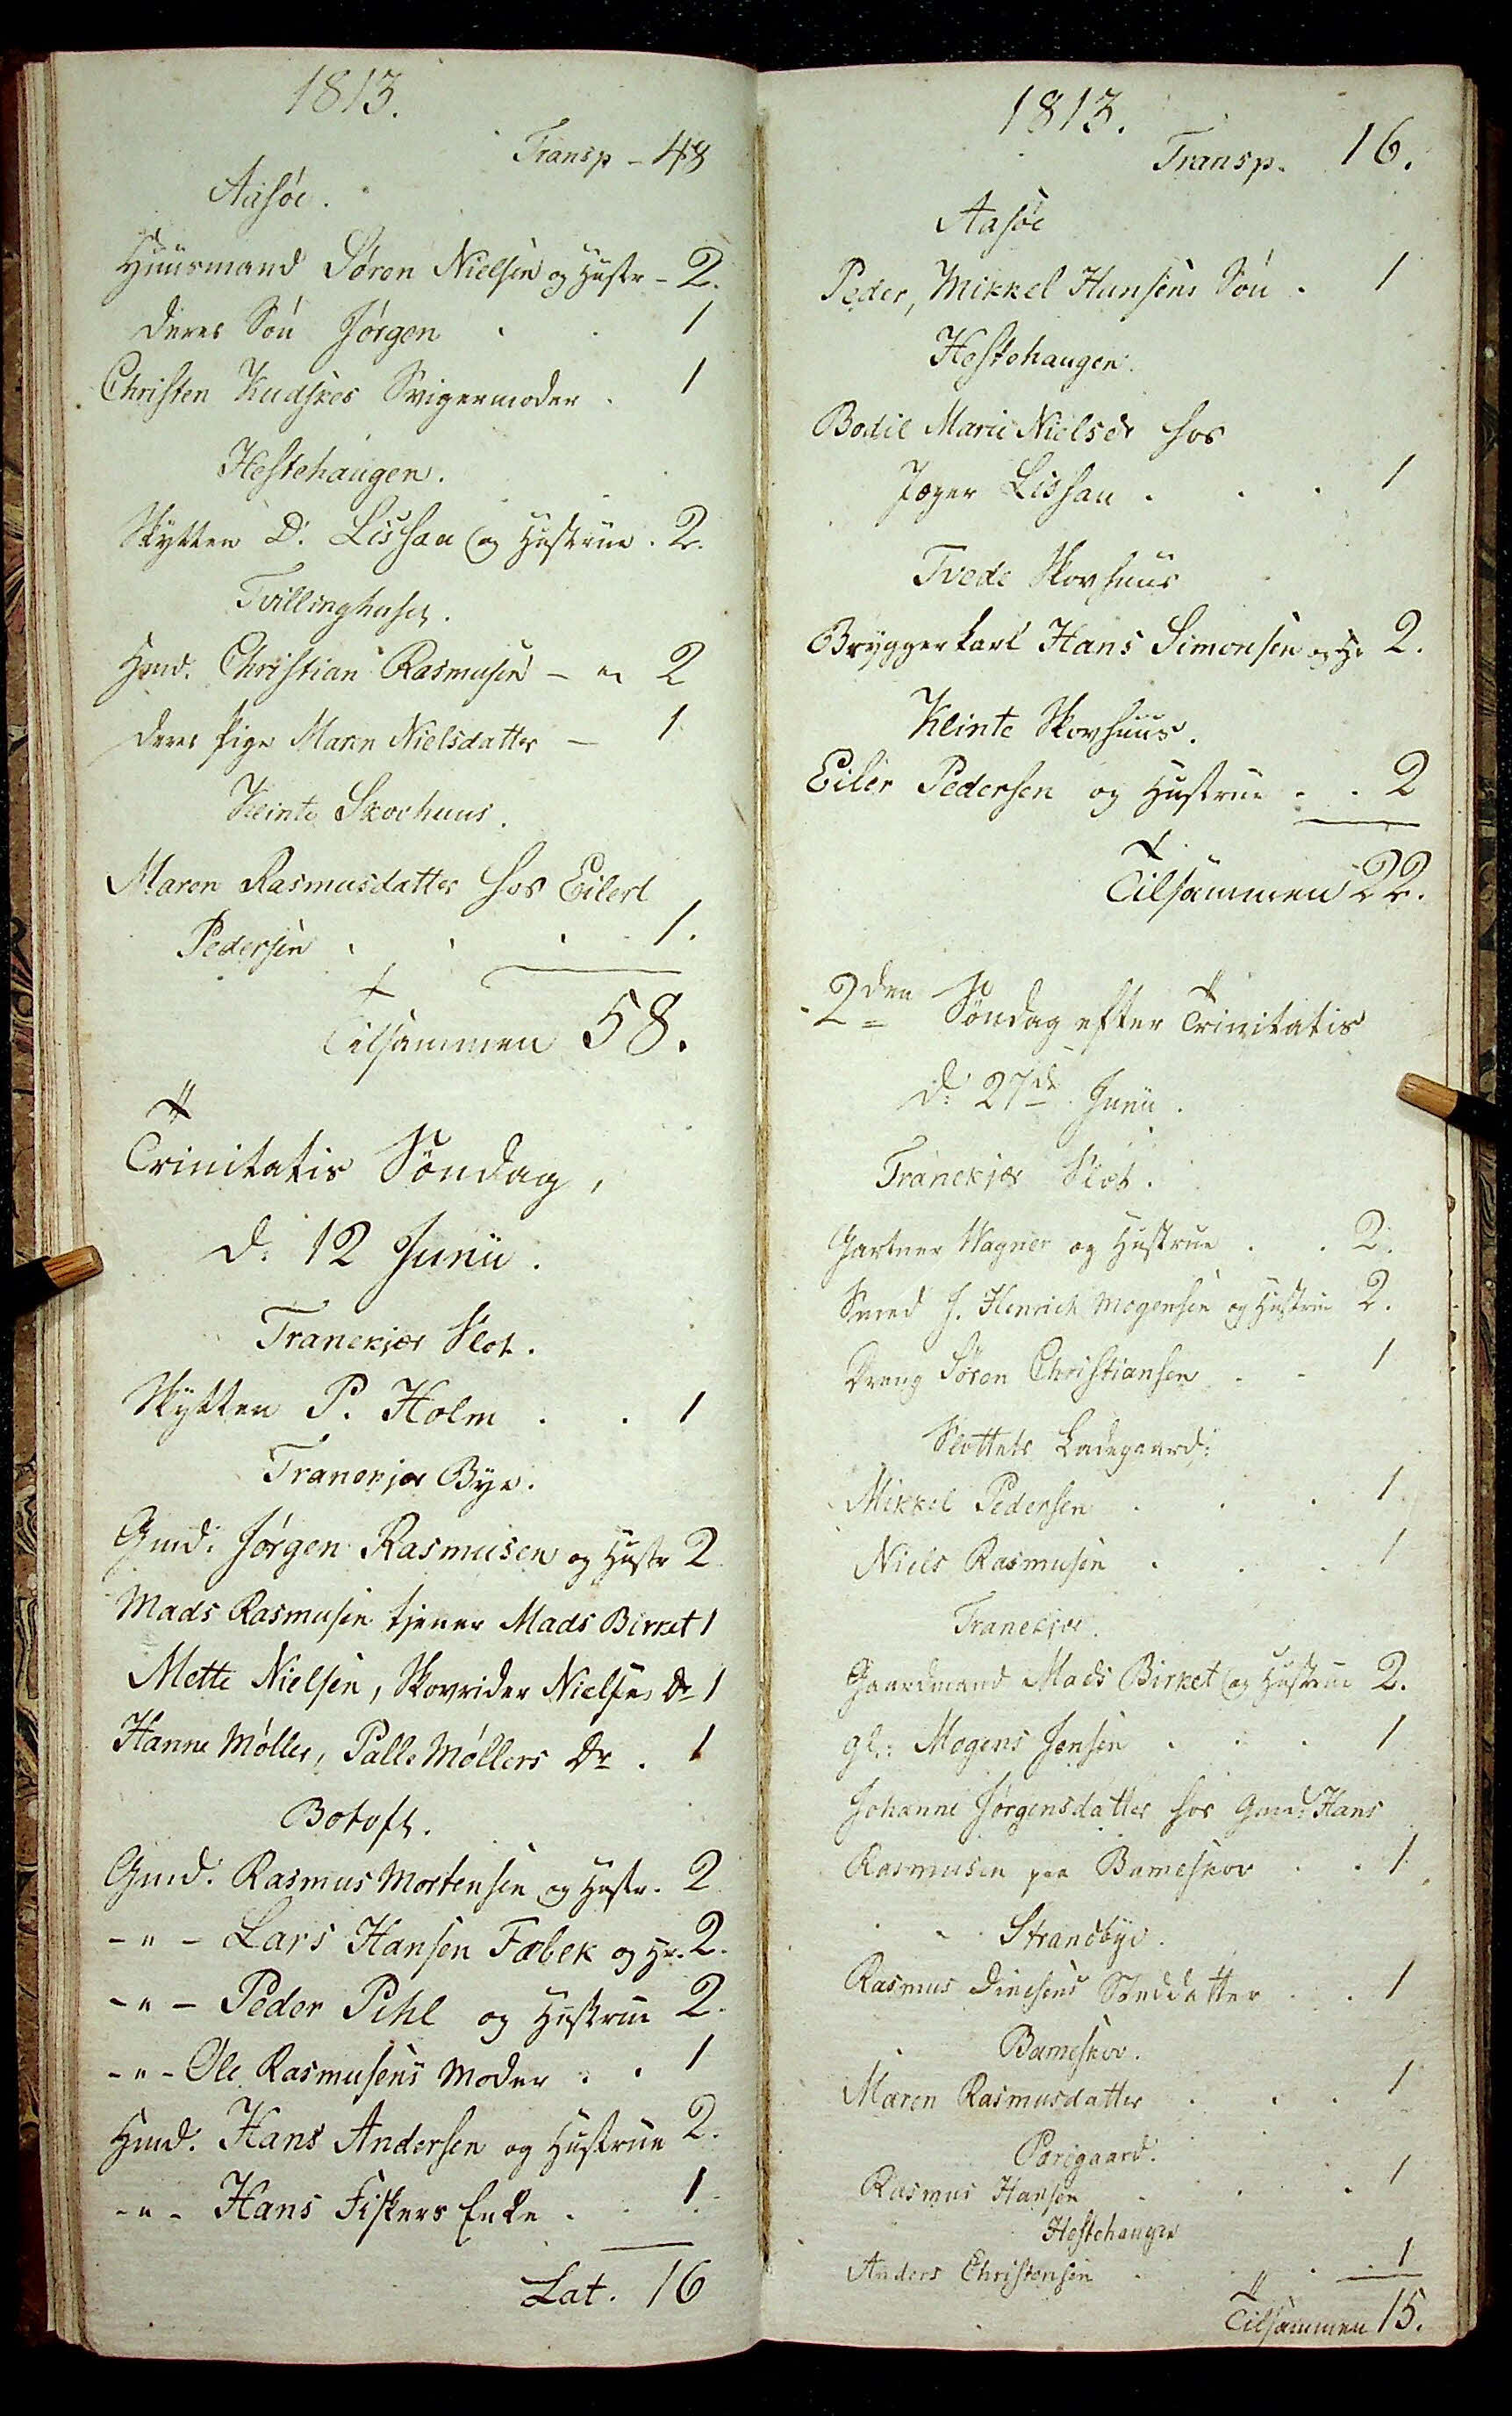1813.
Transp - 48
Aasøe
Huusmand Søren Nielsen og Hustr - 2.
deres Søn Jørgen . . 1
Christen Kudskes Svigermoder . 1
Hestehaugen.
Skytten D. Lissau og Hustrue . 2.
Tvillinghuset.
Hmd. Christian Rasmussen - - 2
deres Pige Maren Nielsdatter - 1
Klinte Skovhuus.
Maren Rasmusdatter hos Eilert
Pedersen . . . . 1.
Tilsammen 58.
Trinitatis Søndag,
d: 12 Junii.
Tranekjær Slot.
Skytten P. Holm . . 1
Tranekjær Bye.
Gmd. Jørgen Rasmusen og Hustr 2.
Mads Rasmusen tjener Mads Birret 1
Mette Nielsen, Skovrider Nielses Dr 1
Hanne Møller, Palle Møllers Dr . 1
Botoft.
Gmd. Rasmus Mortensen og Hustr . 2.
-"- Lars Hansen Fæbek og Hr . 2.
-"- Peder Pihl og Hustrue 2.
-"- Ole Rasmusens Moder . . 1
Hmd. Hans Andersen og Hustrue 2.
-"- Hans Fiskers Enke . . 1.
Lat. 16
1813.
Transp. 16.
Aasøe
Peder, Mikkel Hansens Søn . 1
Hestehaugen.
Bodil Marie Nielsdr hos
Jæger Lissau . . . 1
Tvede Skovhuus
Bryggerkarl Hans Simonsen og Hr 2.
Klinte Skovhuus
Eiler Pedersen og Hustrue . . 2
Tilsammen 22.
2den Søndag efter Trinitatis
d: 27de Junii
Tranekjær Slot.
Gartner Wagner og Hustrue . . 2
Smed J. Henrich Mogensen og Hustrue 2.
Dreng Søren Christiansen . . 1
Slottets Ladegaard:
Mikkel Pedersen . . . 1.
Niels Rasmussen . . . 1
Tranekjær
Gaardmand Mads Birket og Hustrue 2.
gl: Mogens Jensen . . . 1
Johanne Jørgensdatter hos Gmd: Hans
Rasmusen paa Bameskov . . 1
Strandbye.
Rasmus Dinesens Sønddatter . . 1
Bamesskov.
Maren Rasmusdatter . . . 1
Pæregaard.
Rasmus Hansøn . . . 1
Hestehaugen
Anders Christensen . . . . 1
Tilsammen 15.
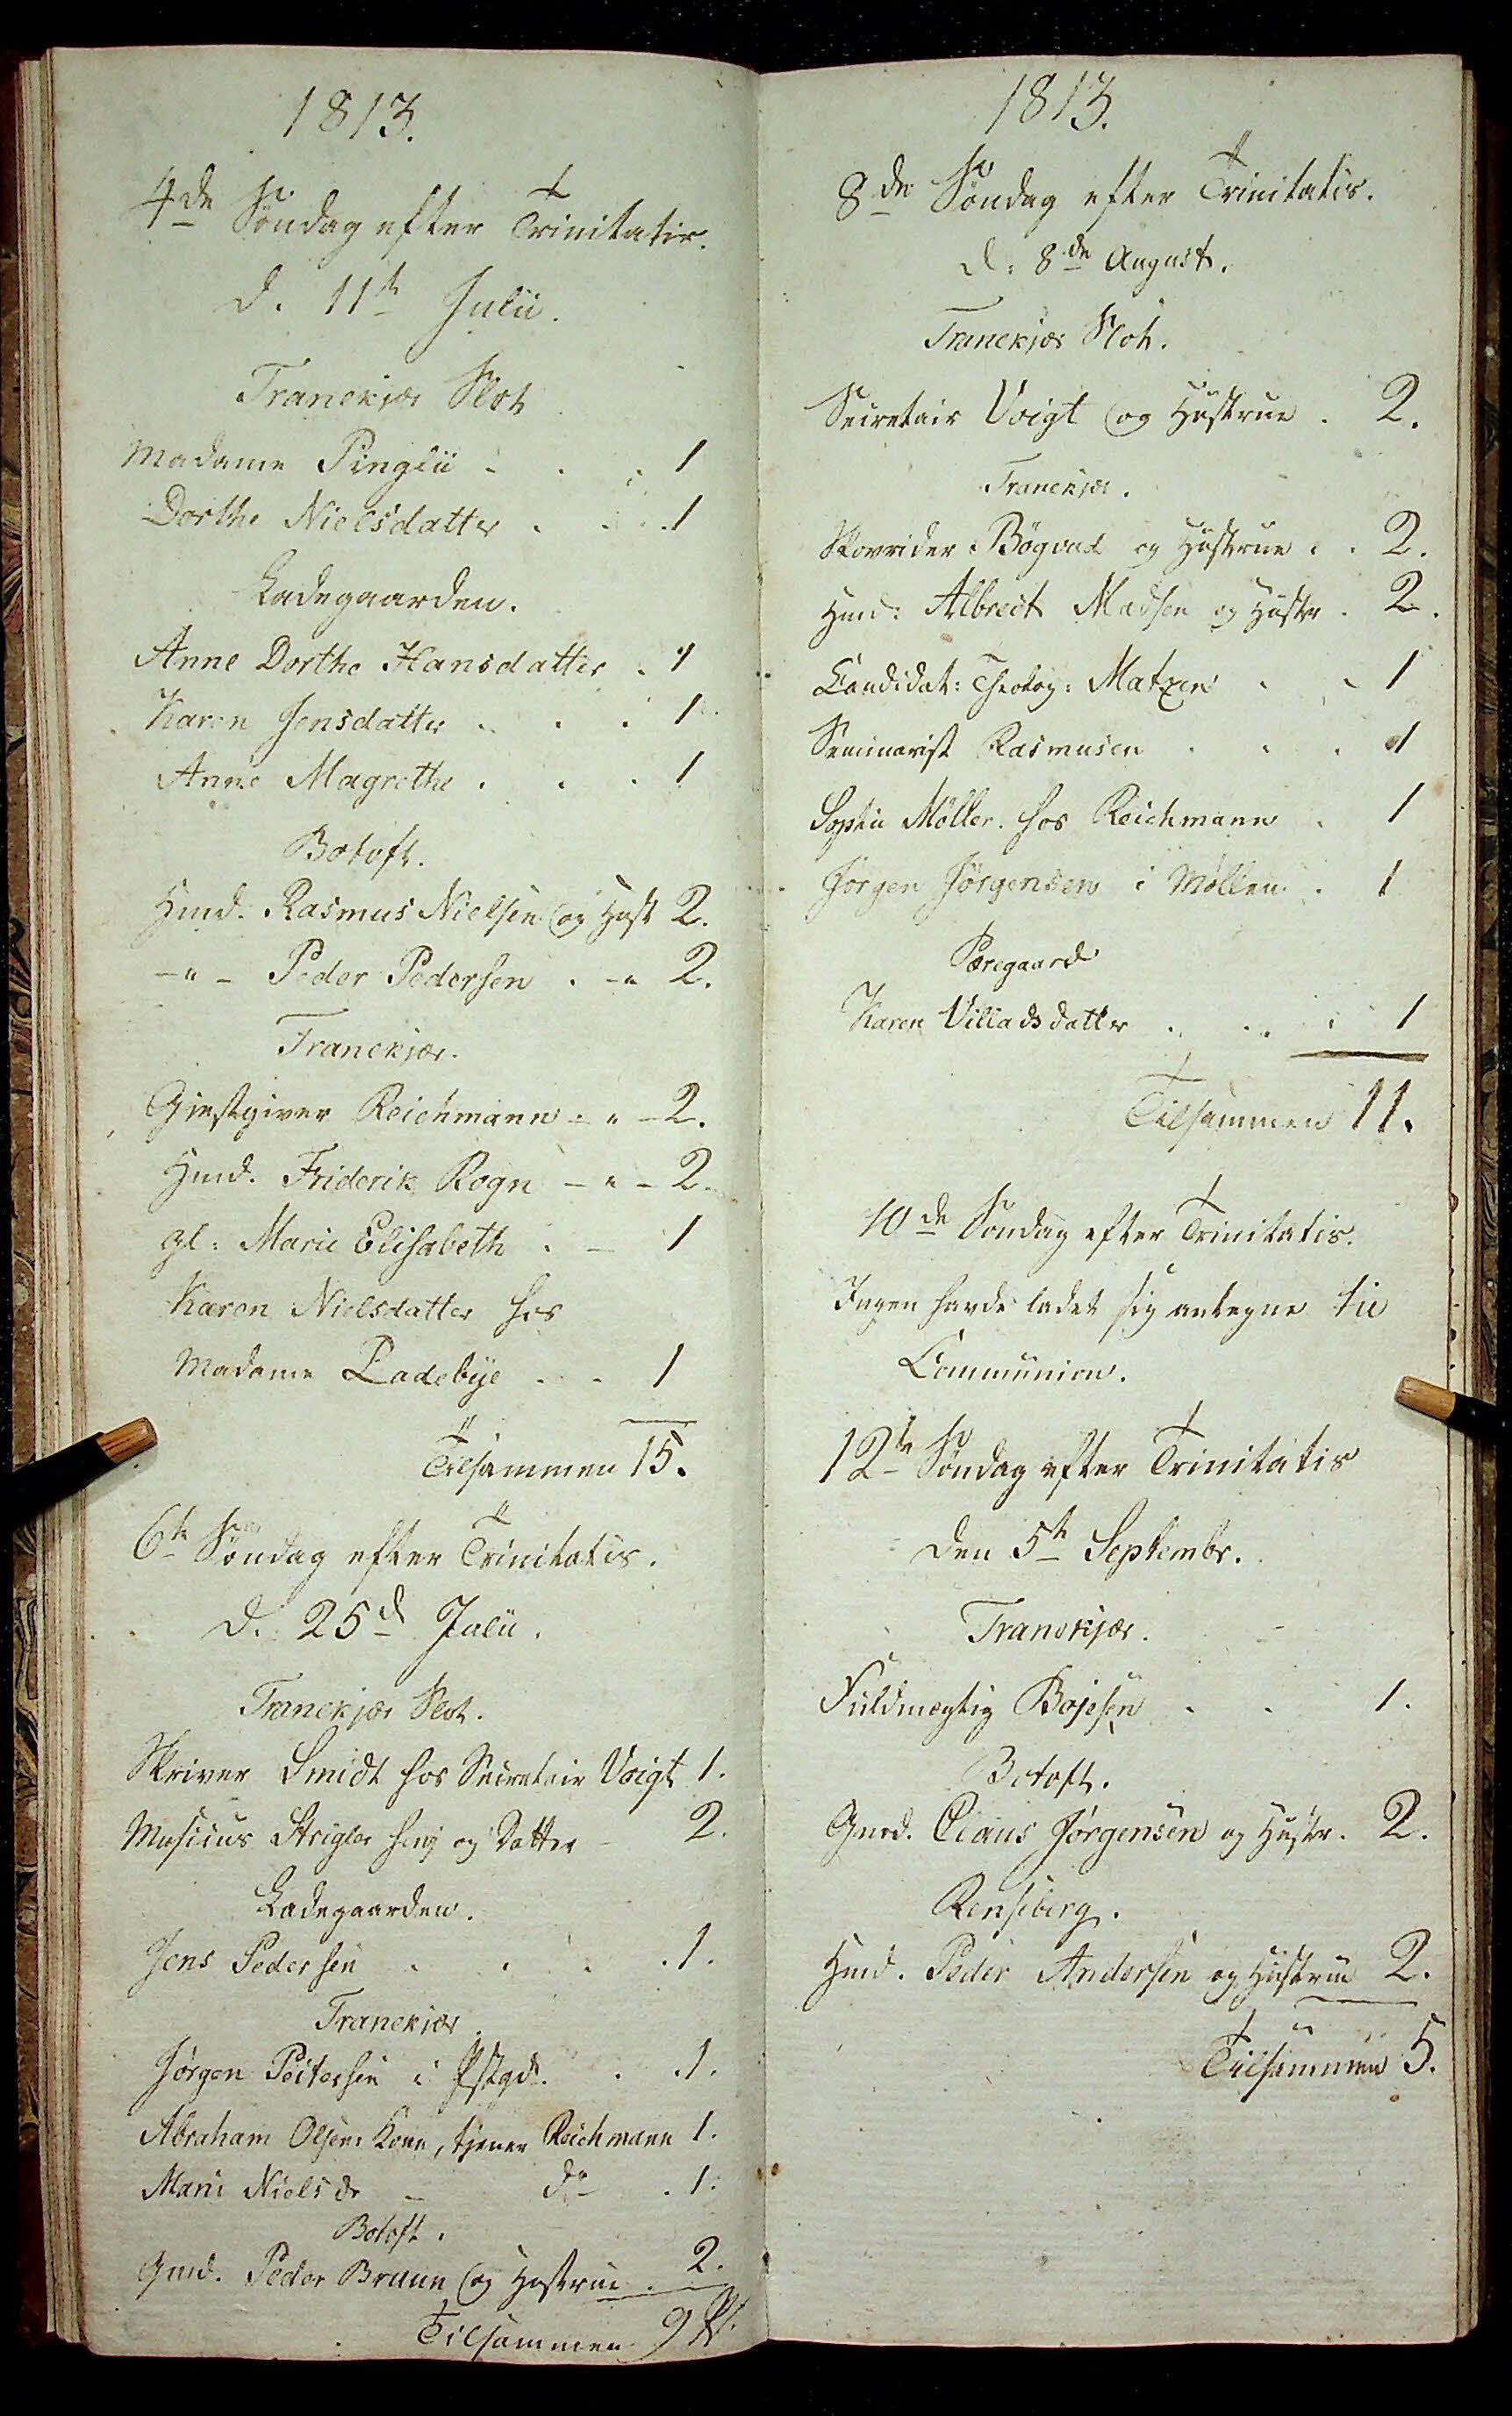1813.
4de Søndag efter Trinitatis.
d. 11te Julii
Tranekjær Slot
Madame Pinglii . . . 1
Dorthe Nielsdatter . . . 1
Ladegaarden.
Anne Dorthe Hansdatter . 1
Karen Jensdatter . . . 1
Anne Magrethe . . . 1
Botoft.
Hmd: Rasmus Nielsen og Hust 2.
-"- Peder Pedersen . -"- 2.
Tranekjær.
Giestgiver Reichmann -"-  2.
Hmd. Friderik Rogn -"- 2.
gl. Marie Elisabeth . - 1
Karen Nielsdatter hos
Madame Ladebye . . 1
Tilsammen 15.
6te Søndag efter Trinitatis.
d. 25de Julii.
Tranekær Slot.
Skriver Smidt hos Secretait Voigt 1
Musicus Strigter H## og Datter - 2
Ladegaarden.
Jens Pedersen . . . . 1.
Tranekjær
Jørgen Peitersen i Pstgd . . . 1.
Abraham Olsens Kone, tiener Reichmann 1.
Marie Nielsdr - do . 1.
Botoft.
Gmd. Peder Bruun og Hustrue . 2.
Tilsammen 9 Ps
1813.
8de Søndag efter Trinitatis.
d. 8de August.
Tranekjær Slot.
Secretair Voigt og Hustrue . 2.
Tranekjær.
Skovrider Bøgvad og Hustrue . . 2.
Hmd: Albrect Madsen og Hustr . 2.
Candidat: Theolog: Matzen . . 1
Seminarist Rasmusen . . . 1
Sophie Møller hos Riechmann . 1
Jørgen Jørgensen i Møllen . 1
Pæregaard
Karen Villadsdatter . . . . 1
Tilsammen 11.
10de Søndag efter Trinitatis.
Ingen havde ladet sig antagne til
Communion.
12te Søndag efter Trinitatis.
Den 5te Septembr.
Tranekjær.
Fuldmægtig Bojesen . . 1.
Botoft.
Gmd. Claus Jørgensen og Hustr . 2.
Renseberg
Hmd. Peder Andersen og Hustrue 2.
Tilsammen 5.